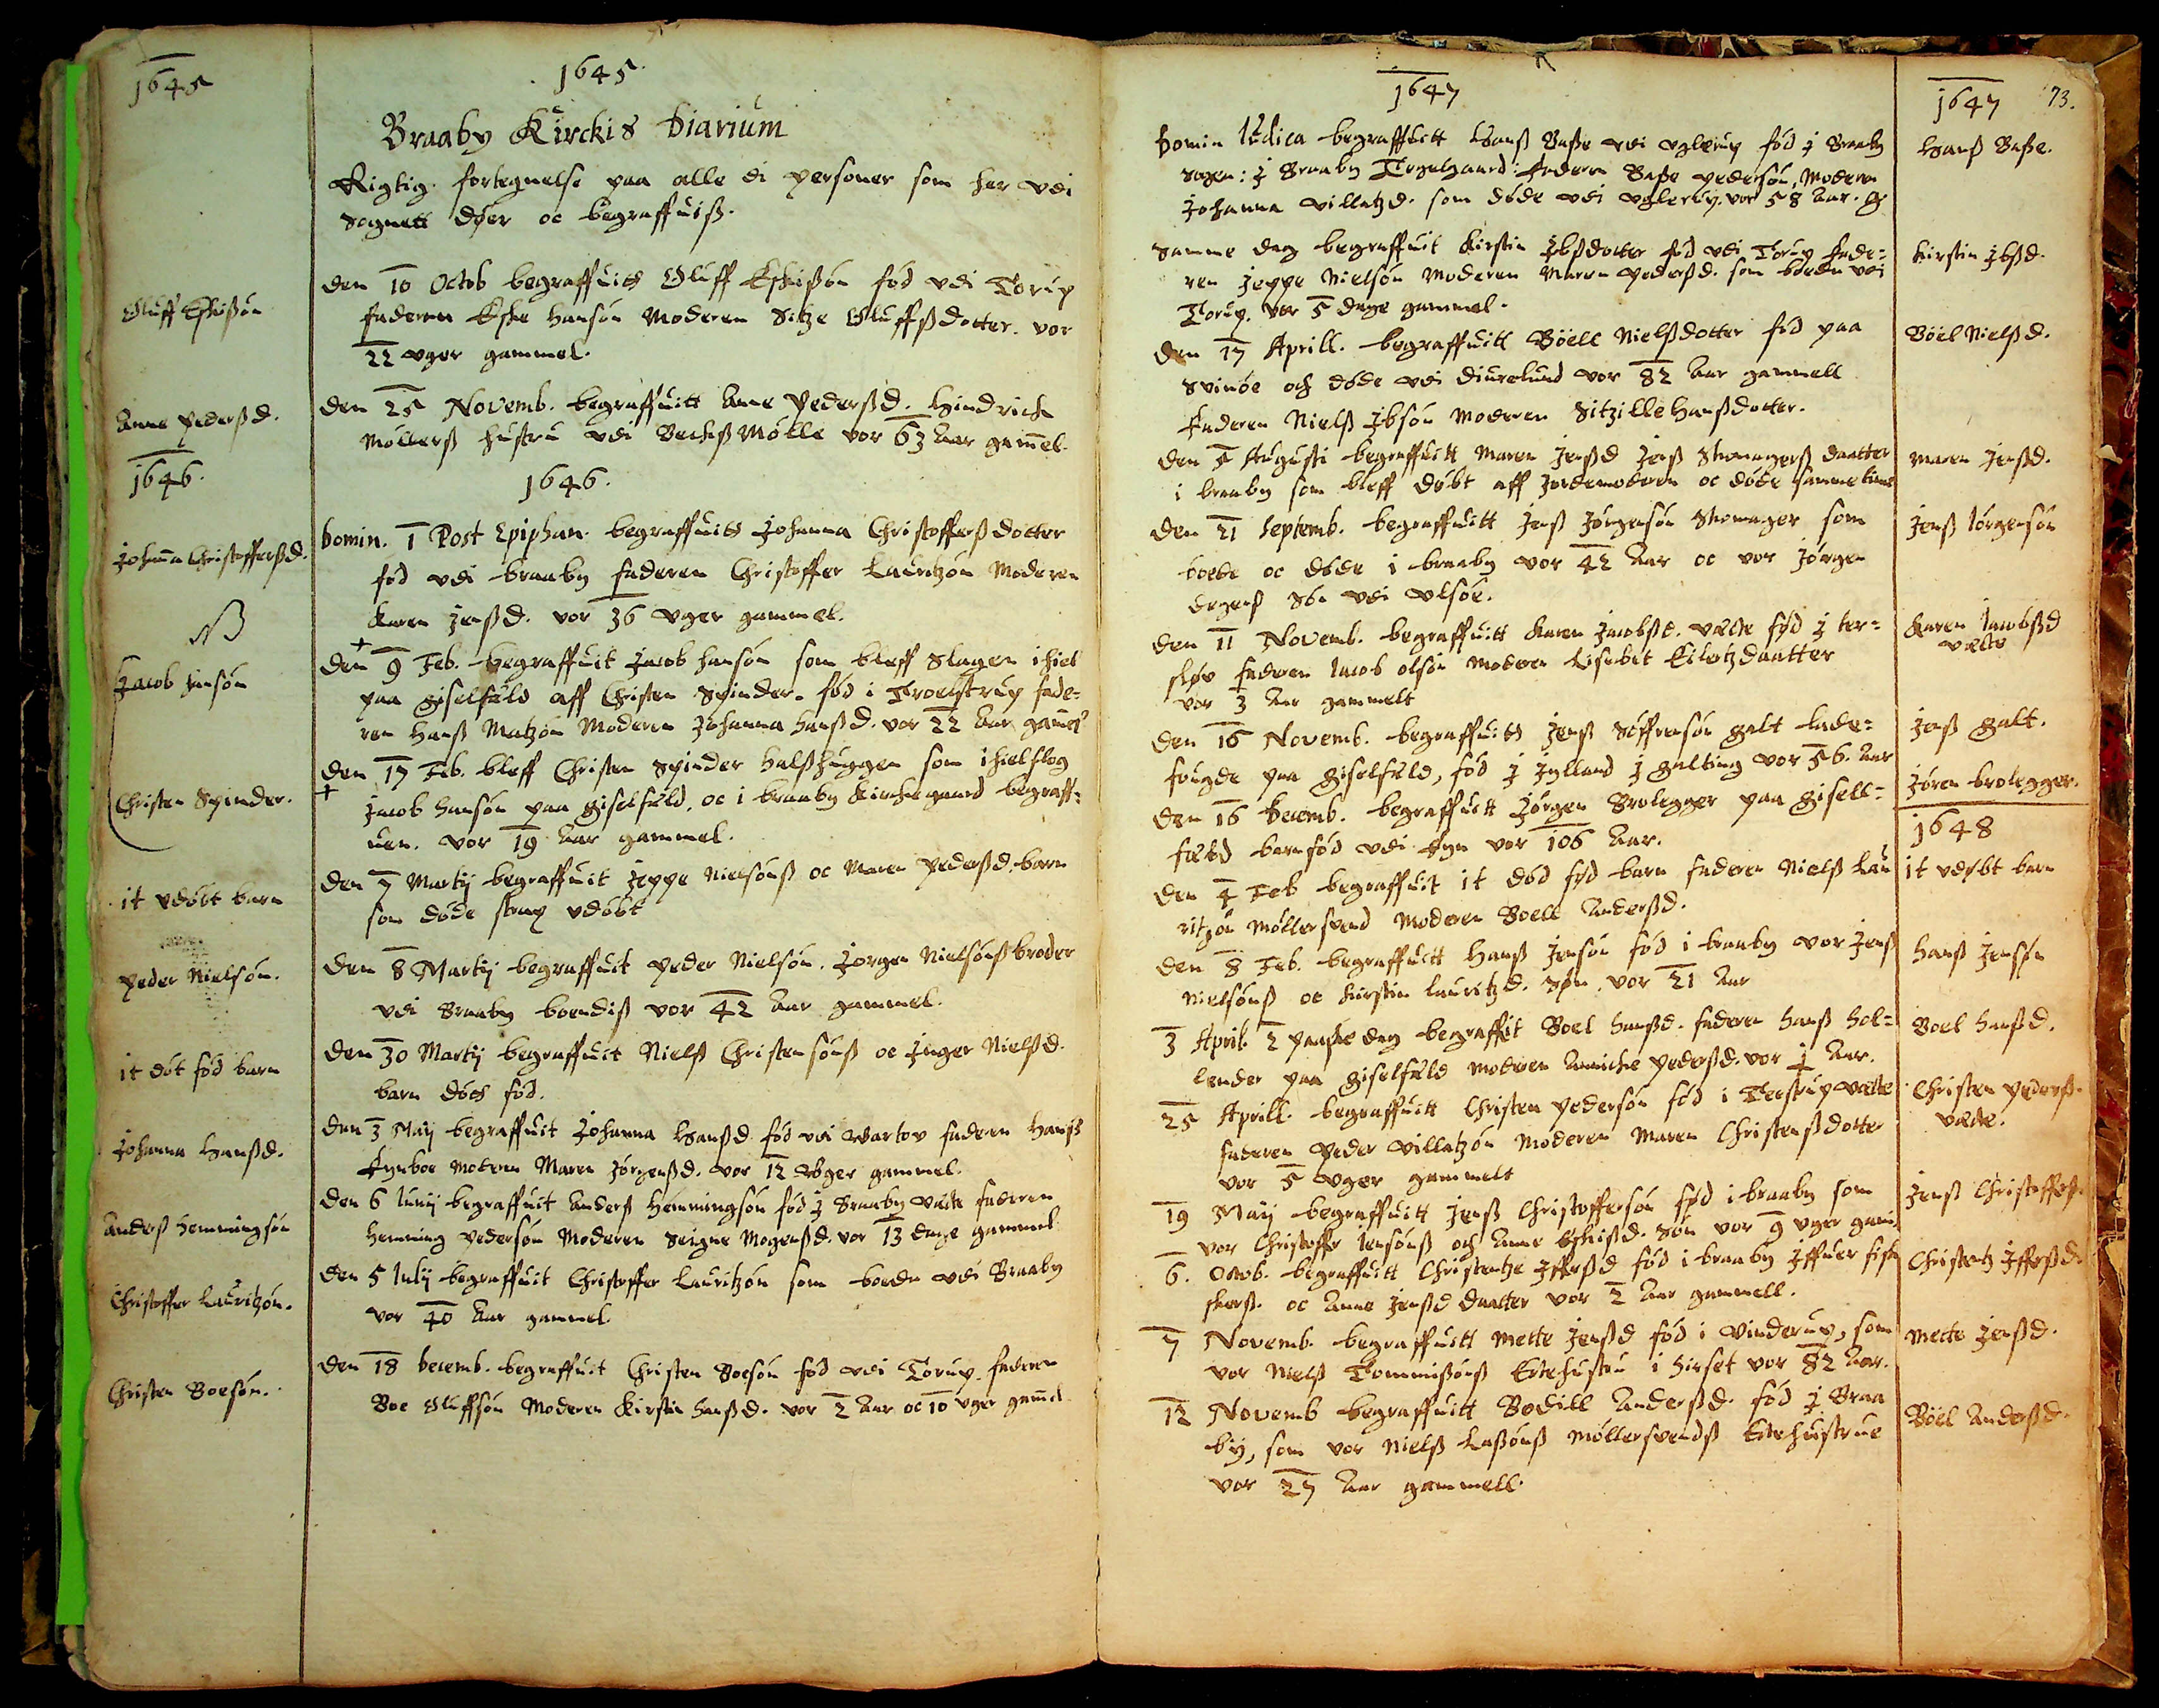1645 
1645
Braaby Kirckis Diarium
Rigtig fortegnelse paa alle di personer som her udi
sognett døer oc begraffuis 
Oluff Eskisøn 
Den 10 Octob begraffuitt Oluff Eskisøn fød Udi Torup
Faderen Eske Hansøn, Moderen Sitze Oluffsdatter. var
22 Uger gammel. 
Anna Pedersd.
Den 25 Novemb. begraffuitt Anna Pedersd. Hindrich
Møllers hustru Udi Bechsmølle var 63 Aar gammel.
1646
Johanna Christofferd.
1646
Domin.1. Post Epiphan. begraffuitt Johanna Christoffersdotter
fød Udi Braaby Faderen Christoffer Lauritzøn Moderen
Karen Jensd. var 36 Uger gammel.
NB
Jacob Hansøn
Den 9 Feb. begraffuit Jacob Hansøn, som bleff slagen ihiel
paa Giselfeld aff Christen Spinder. Fød i Troelstrup Fade¬
ren Hans Matzøn Moderen Johanna Hansd. var 22 Aar gammel.
Christen Spinder.
Den 17 Febr. bleff Christen Spinder halshuggen som ihielslog
Jacob Hansøn paa Giselfeld, oc i Braaby Kirchegaard begraff¬
en. var 19 Aar gammel. 
it udøbt barn 
Den 7 Marty begraffuit Jeppe Nielsøns oc Maren Pedersd barn
som døde strax udøbt.
Peder Nielsøn
Den 8 Marty begraffuit Peder Nielsøn. Jørgen Nielsøns broder
Udi Braaby boendis var 42 Aar gammel.
it død fød barn
Den 30 Marty begraffuit Niels Christensøns oc Jnger Nielsd.
barn død fød.
Johanna Hansd.
Den 3 May begraffuit Johanna Hansd. fød udi Wartov Faderen Hans
Fynboe## Moderen Maren Jørgensd. var 12 Uger gammel.
Anders Hemmingsøn 
Den 6 Juny begraffuit Anders Hemmingsøn fød J Braaby Mark Faderen
Hemming Pedersøn Moderen Seigne Mogensd. var 13 Dage gammel.
Christoffer Lauritzøn 
Den 5 July begraffuit Christoffer Lauritzøn som boede udi Braaby
var 40 Aar gammel. 
Christen Boesøn
Den 18. Decemb. begraffuit Christen Boesøn fød udi Torup. Faderen
Boe Oluffsøn Moderen Kirsten Hansd. var 2 Aar oc 10 Uger gammel.
1647 
Domin Judica begraffuitt Hans Base udi Uglerup fød J Braaby
Sogn: J Braaby Tegelgaard: Faderen Base Pedersøn, Moderen
Johanne Villatzd. som døde Udi Uglerup var 58 Aar g.
73.
1647
Hans Base.
Samme dag begraffuit Kirstin Jbsdotter fød udi Torup Fade¬
ren Jeppe Nielsøn Moderen Maren Pedersd. som boede udi
Torup var 5 Dage gammel.
Kirstin Jbsd.
Den 17. Aprill begraffuitt Boell Nielsdotter fød paa
Svinøe## och døde udi Diurelund var 82 Aar gammel
Faderen Niels Jbsøn Moderen Sitzille Hansdotter.
Boel Nielsd.
Den 4. Augusti begraffuitt Maren Jensd Jens Skomagers Daatter
i Braaby som bleff døbt aff Jordemoderen oc døde samme time.
Maren Jensd.
Den 21 Septemb. begraffuitt Jens Jørgensøn Skomager som
boede oc døde i Braaby var 42 Aar oc var Jørgen
Degnes Søn udi Ulsøe.
Jens Jørgensøn
Den 11 Novemb. begraffuitt Karen Jacobsd. uæcte fød J Tær¬
sløv## Faderen Jacob Olsøn Moderen Lisebet Eilertzdaatter
var 3 Aar gammel.
Karen Jacobsd.
uæcte
Den 16. Novemb. begraffuitt Jens Søffrensøn Galt Lade¬
fougde paa Giselfeld fød J Jylland J Gelting var 56 Aar.
Jens Galt.
Den 16 Decemb. begraffuitt Jørgen Brolegger paa Gisell¬
fæld barnfød udi Fyn var 106 Aar
Jøren Brolegger.
1648
Den 4 Feb. begraffuit it død fød barn Faderen Niels Lau
ritzøn Møllersvend Moderen Boell Andersd.
it udøbt barn
Den 8 Feb. begraffuitt Hans Jensøn fød i Braaby var Jens
Nielsøns oc Kirstin Lauritzd. Søn var 21 Aar.
Hans Jensøn
3 April. 2 Paaskedag begraffit Boel Hansd. Faderen Hans Hol¬
lænder paa Giselfæld Moderen Anniche Pedersd. var  ½ Aar.
Boel Hansd.
25 Aprill. begraffuitt Christen Pedersøn fød i Teestrup uæcte
Faderen Peder Villatzøn Moderen Maren Christensdatter
var 5 Uger gammelt.
Christen Peders.
uæcte 
19. May begraffuitt Jens Christoffersøn fød i Braaby som
var Christoffer Jensøns och Anna Eshisd.## Søn var 9 Uger gam##
Jens Christoffers.
6. Octb begraffuitt Christentze Jffersd. fød i Braaby Iffuer Fisc##
hers## oc Anne Jensd Daatter var 2 Aar gammell.
Christentz## Jffersd.
7 Novemb. begraffuitt Mette Jensd fød i Vinderup, som
var Niels Tommisøns Ectehustrue## i Hirset var 82 Aar.
Mette Jensd.
12 Novemb. begraffuitt Bodill Andersd. fød J Braa
by, som var Niels Lassøns Møllersvends Ectehustrue##
var 27 Aar gammell. 
Boel Andersd.
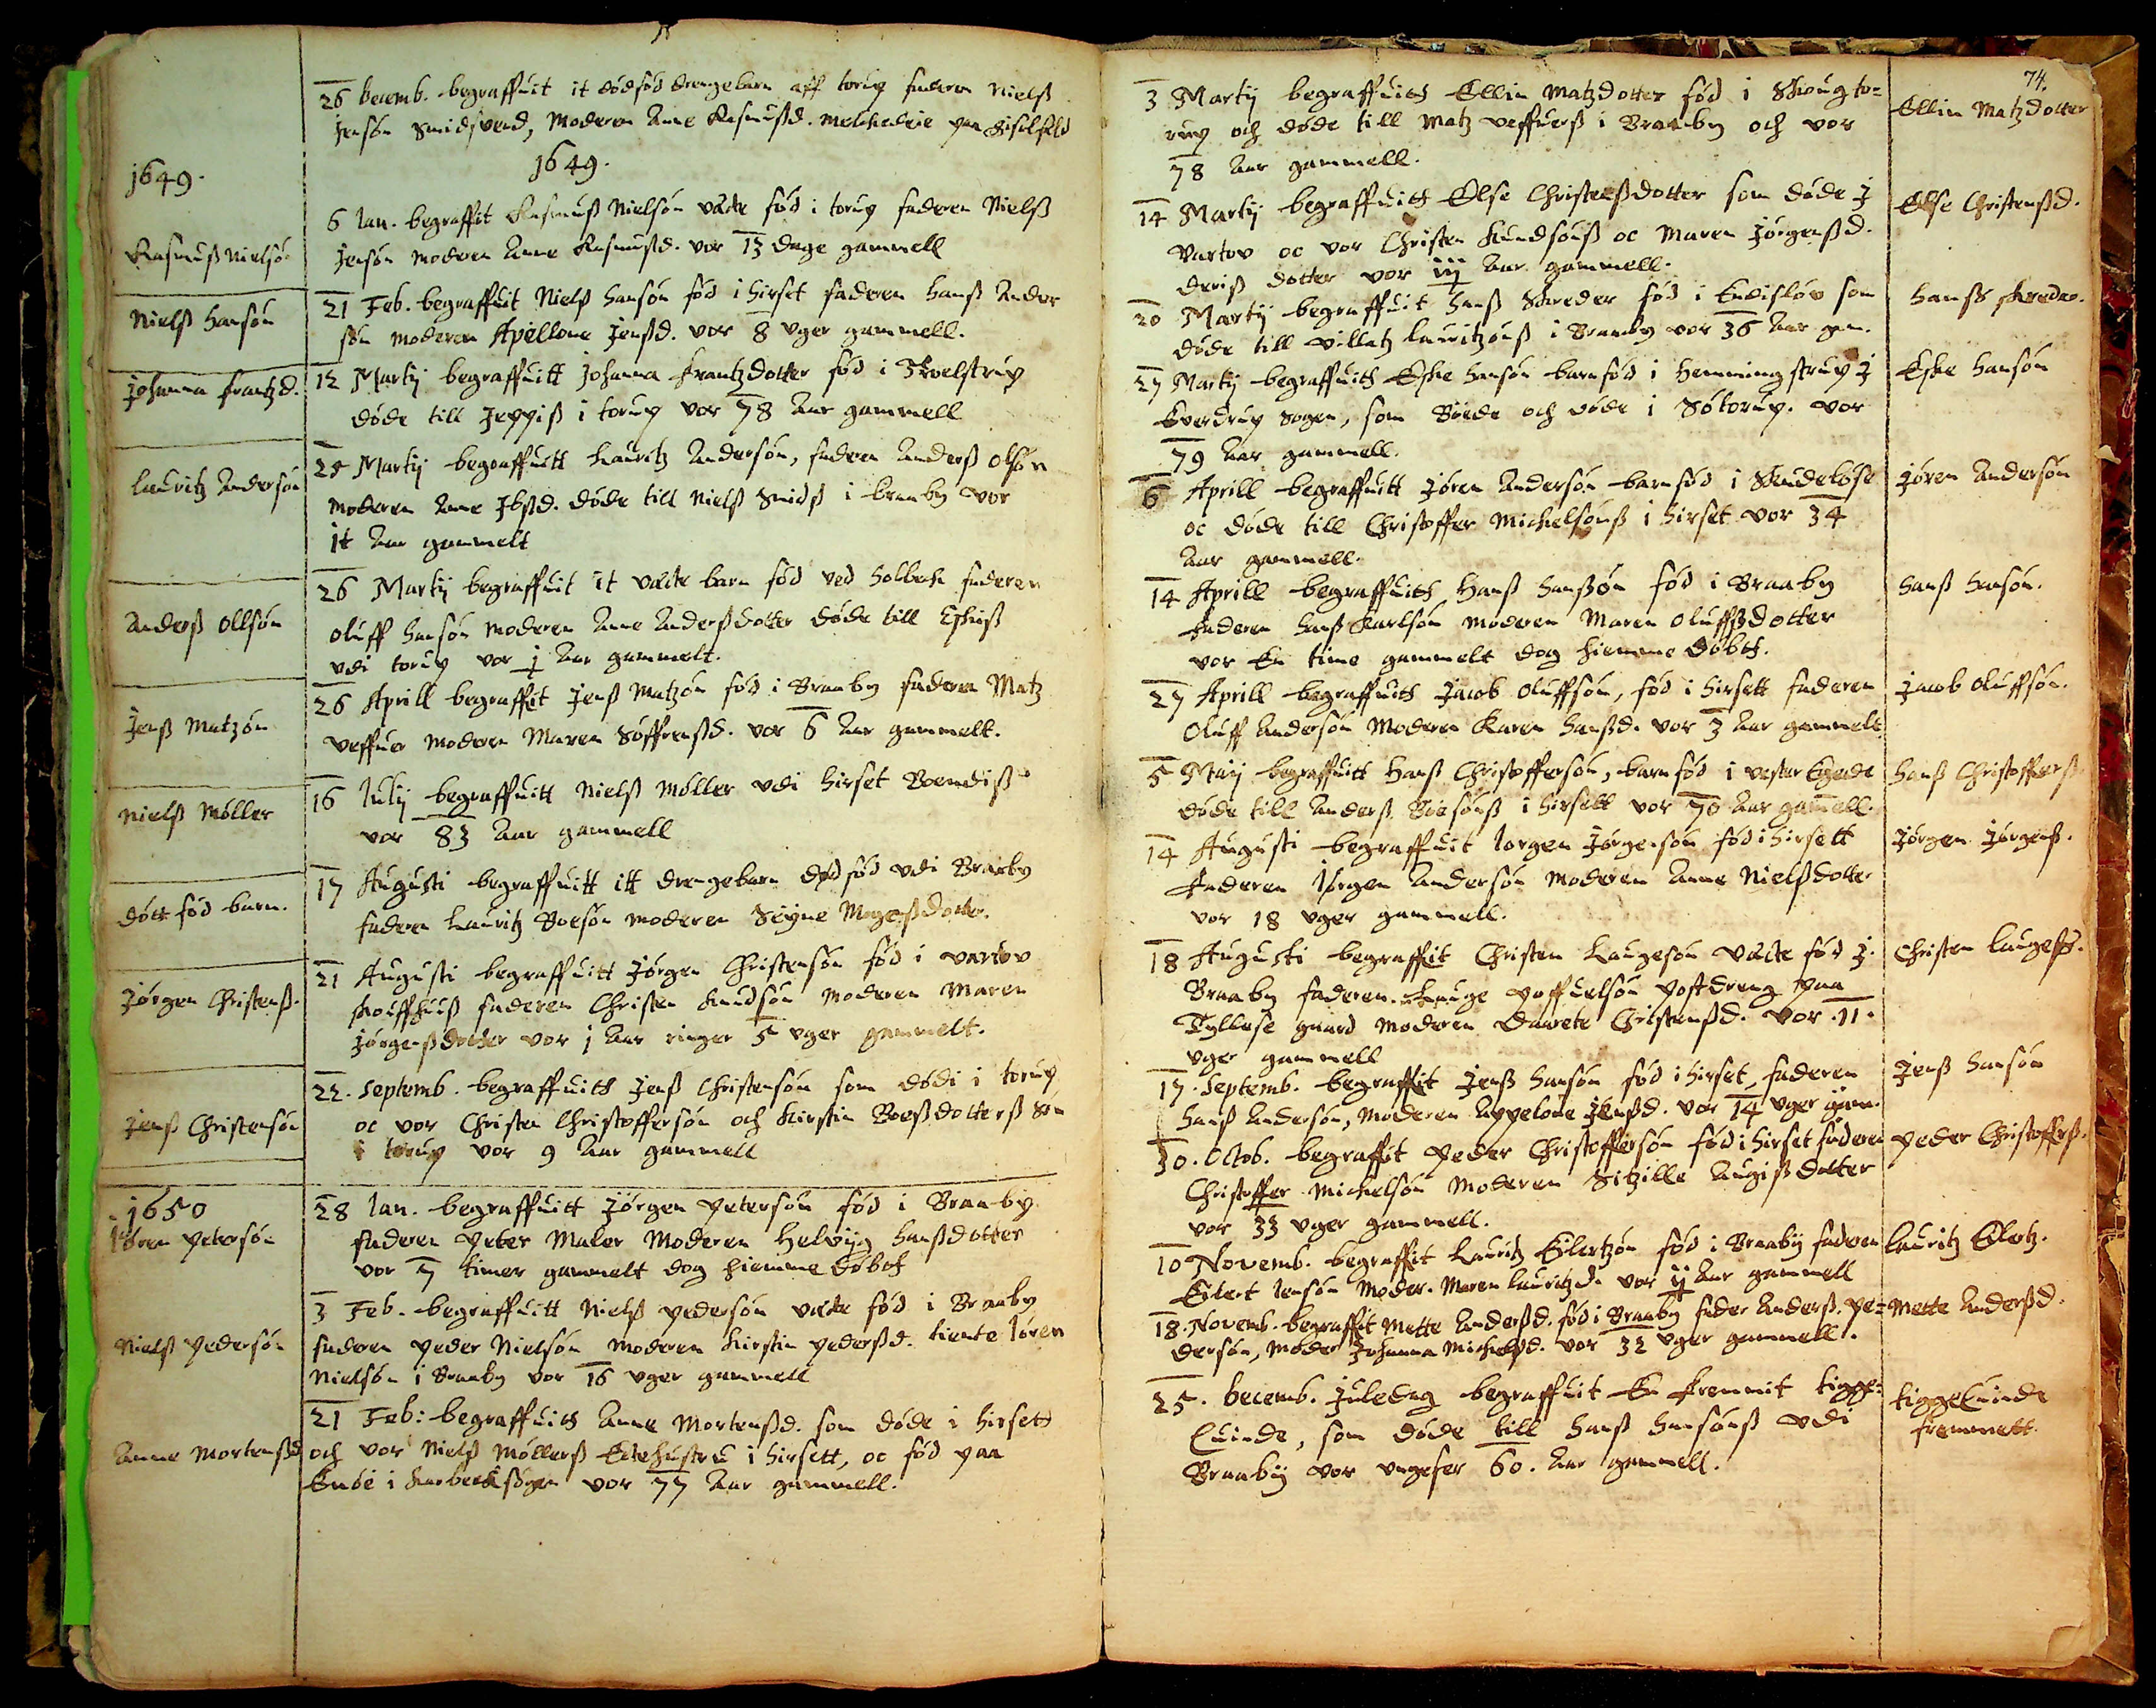26. Decemb. begraffuit it dødfød drengebarn aff Torup Faderen Niels
Jensøn Smidssvend, Moderen Anne Rasmusd. Malchedie## paa Giselfeld.
1649
Rasmus Nielsøn
1649
6 Jan. begraffit Rasmus Nielsøn uæcte fød i Torup Faderen Niels
Jensøn Moderen Anne Rasmusd. var 13 Dage gammell.
Niels Hansøn
21 Feb. begraffuit Niels Hansøn fød i Hirset Faderen Hans Ander
søn Moderen Apellone Jensd. var 8 Uger gammell.
Johanne Frantzd.
12. Marty begraffuitt Johanne Frantzdatter fød i Thoelstrup
døde till Jeppis i Torup var 78 Aar gammell.
Lauritz Andersøn
24. Marty begraffuitt Lauritz Andersøn, Faderen Anders Olsøn
Moderen Ane Jbsd. døde till Niels Smids i Braaby var
it Aar gammell. 
Anders Ollsøn 
26 Marty begraffuit it uæcte barn fød ved Holbech, Faderen
Oluff Hansøn, Moderen Anne Andersdatter døde til Eskis##
udi Torup, var ½ Aar gammelt.
Jens Matzøn 
26. Aprill begraffit Jens Matzøn fød i Braaby Faderen Matz
Veffuer Moderen Maren Søffrensd. var 6 Aar gammelt.
Niels Møller
16. July begraffuit Niels Møller udi Hirset Boendis
var 83 Aar gammell. 
Død fød barn
17. Augusti begraffuitt itt Drengebarn dødfød udi Braaby
Faderen Lauritz Boesøn Moderen Seigne Mogensdatter.
Jørgen Christens.
21. Augusti begraffuitt Jørgen Christensøn fød i Vartov
Skouffhuus Faderen Christen Knudsøn, Moderen Maren
Jørgensdatter var 1 Aar ringer 5 Uger gammelt.
Jens Christensøn
22. Septemb. begraffuitt Jens Christensøn som dødi i Torup
oc var Christen Christoffersøn och Kirstin Boesdatters Søn
i Torup var 9 Aar gammell.
1650
Jørgen Pedersøn
28 Jan. begraffuit Jørgen Pedersøn fød i Braaby
Faderen Peder Maler Moderen Helviig Hansdatter
var 7 timer gammelt dog hjemmedøbet.
Niels Pedersøn
3. Feb. begraffuitt Niels Pedersøn uecte fød i Braaby
Faderen Peder Nielsøn Moderen Kirstin Pedersd. tiente Jøren
Nielsøn i Braaby var 16 Uger gammell. 
Anne Mortensd.
21 Febr: begraffuitt Anne Mortensd. som døde i Hirsett
och var Niels Møllers Ectehustrue i Hirsett, oc fød på
## i Herbeck## Sogn var 77 Aar gammell.
3 Marty begraffuitt Ellin Matzdotter fød i Skougtoe¬
rup och døde till Matz Veffuers i Braaby och var
78 Aar gammell. 
74.
Ellin Matzdotter
14 Marty begraffuitt Else Christensdatter som døde J
Vartov oc var Christen Knudsøns oc Maren Jørgensd.
deris dotter var 2½ Aar gammell.
Else Christensd. 
20 Marty begraffuit Hans Skreder fød i Endisløv som
døde till Villatz Lauritzøns i Braaby var 36 Aar gm.
Hans Skreder
27 Marty begraffuitts Eske Hansøn barnfød i Hemmingstrup J
Everdrup Sogn, som Boede och døde i Søtorup. var
79 Aar gammell. 
Eske Hansøn
6 Aprill begraffuitt Jøren Andersøn barnfød i Skudeløse
oc døde till Christoffer Michelsøns i Hirset var 34
Aar gammell.
Jøren Andersøn
14 Aprill begraffuitts Hans Hansøn fød i Braaby
Faderen Hans Karlsøn, Moderen Maren Oluffsdotter
var En Time gammelt dog hiemmedøbet.
Hans Hansøn
27 Aprill begraffuitts Jacob Oluffsøn, fød i Hirsett Faderen
Oluff Andersøn Moderen Karen Hansd. var 3 Aar gammell.
Jacob Oluffsøn.
5 May begraffuitt Hans Christoffersøn, barnfød i Vester Egede
døde till Anders Boesøns i Hirsett var 70 Aar gammell.
Hans Christoffers
14 Augusti begraffuit Jørgen Jørgensøn fød i Hirsett
Faderen Jørgen Andersøn Moderen Anna Nielsdotter
var 18 Uger gammell.
Jørgen Jørgens.
18 Augusti begraffit Christen Laugesøn uæcte fød J
Braaby Faderen Lauge Poffuelsøn Postdreng på
Tylløse## gaard Moderen Dorete Christensd. var 11
Uger gammell.
Christen Lauges.
17. Septemb. begraffuit Jens Hansøn fød i Hirset, Faderen
Hans Andersen, Moderen Appelone Jensd. var 14 Uger gam##
Jens Hansøn
30 Octob. begraffit Peder Christoffersøn fød i Hirset Faderen
Christoffer Michelsøn Moderen Sitzille Augisdatter
var 33 Uger gammell.
Peder Christoffers.
10. Novemb. begraffit Lauritz Eilertzøn fød i Braaby Faderen
Eilert Jenssøn## Moder. Maren Lauritzd. var 1½ Aar gammell.
Lauritz Eilertz##
18. Novemb. begraffit Mette Andersd. fød i Braaby Fader Anders Pe¬
dersøn Moder Johanne Michelsd. var 32 Uger gammell.
Mette Andersd.
25. Decemb. Juledag begraffuit En fremmit tigge¬
Quinde, som døde till Hans Hansøns udi
Braaby var ungefer 60 Aar gammell.
Tigge Quinde
fremmett.